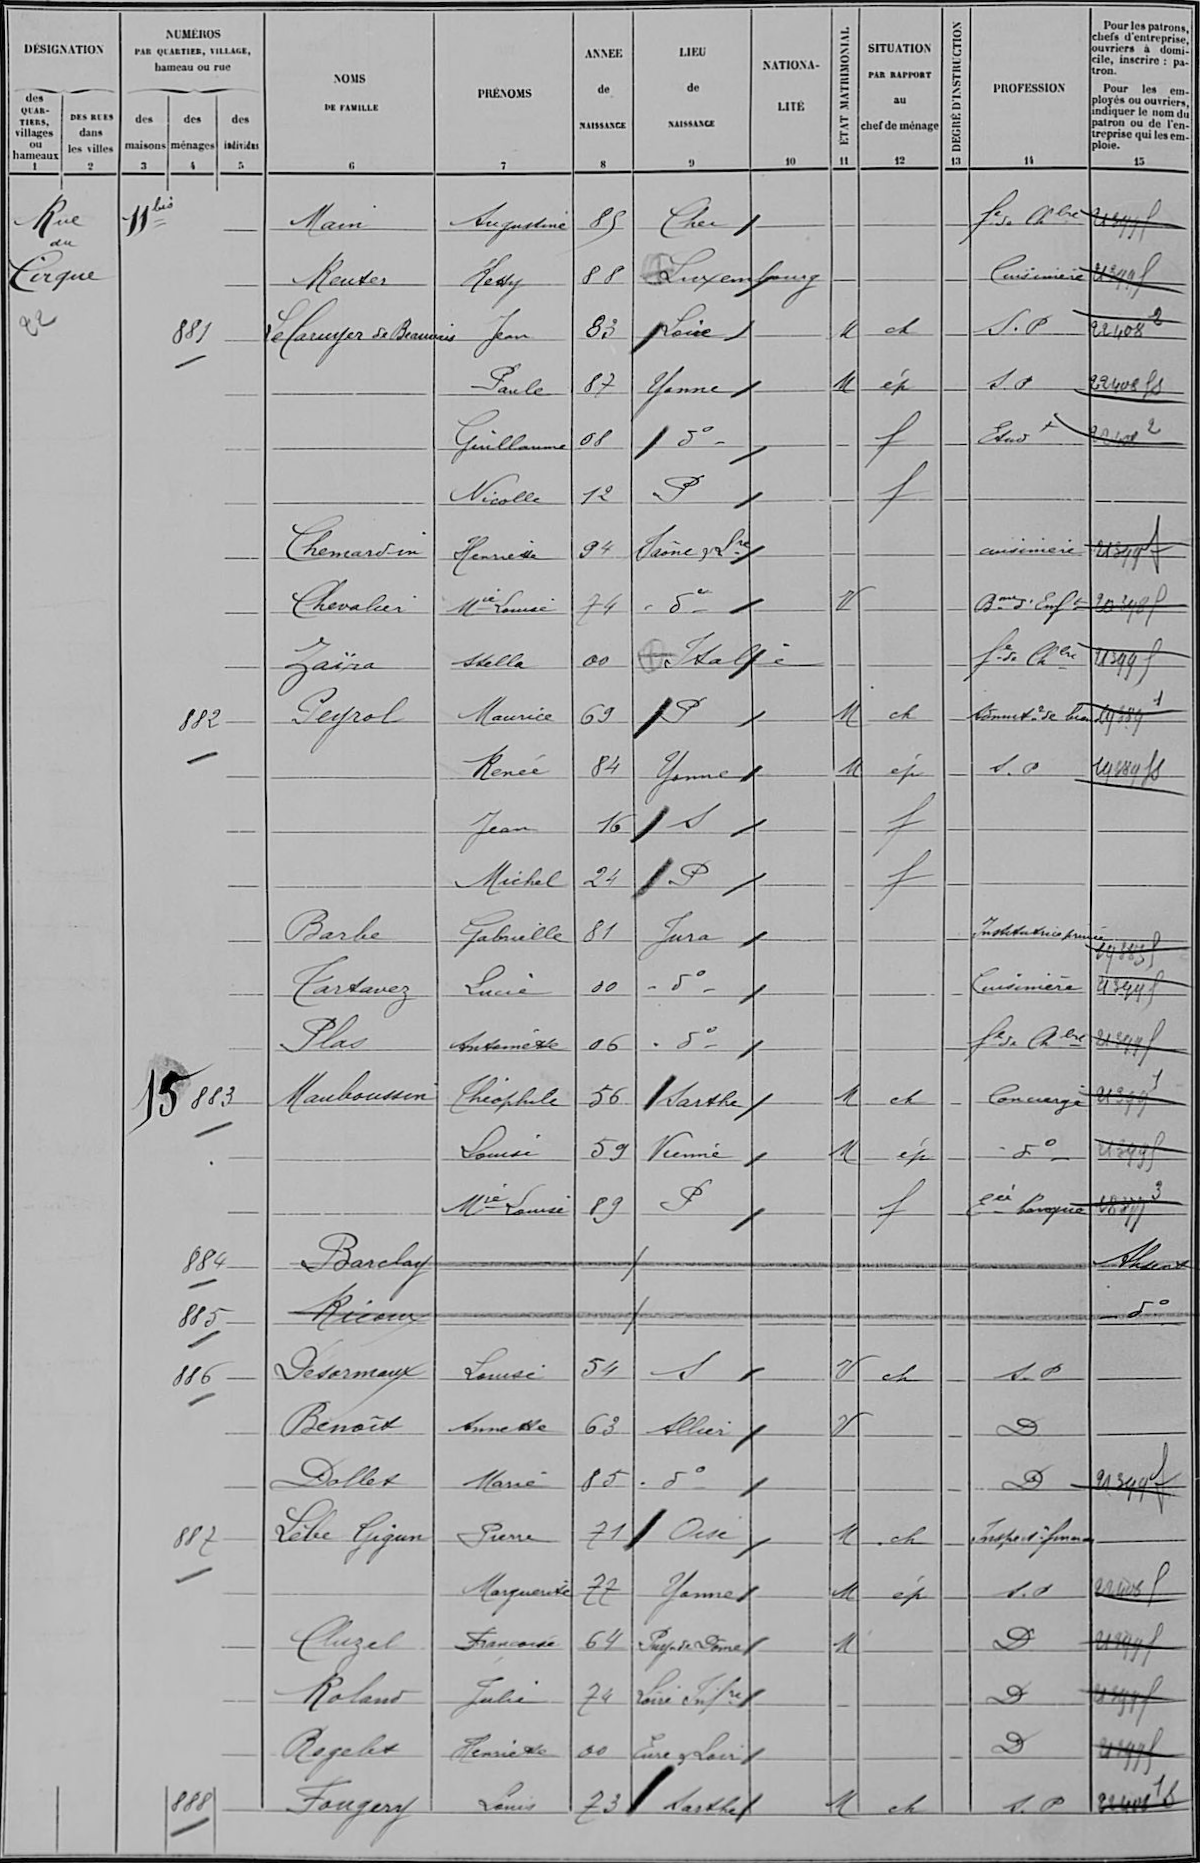Main/Augustine/85/Cher/¤/¤/¤/¤/fe de Chbre/21399 f
Reuter/Kessy/88/Luxembourg/¤/¤/¤/¤/Cuisinière/21399
Le Caruye de Beauvais/Jean/83/Loire/¤/M/ch/¤/S P/22408
¤/Paule/87/Yonne/¤/M/ép/¤/S P/22408 fs
¤/Guillaume/08/S°/¤/¤/f/¤/Etudt/22408
¤/Nicolle/12/P/¤/¤/f/¤/¤/¤
Chemardin/Henriette/94/Saône et Lre/¤/¤/¤/¤/cuisinière/21399 f
Chevalier/Mie Louise/74/d°/¤/V/¤/¤/Bne d'Enft/20348 f
Zaïra/Stella/00/Italie/¤/¤/¤/¤/fe de Chbre/21399 f
Peyrol/Maurice/69/P/¤/M/ch/¤/Bonnetr de bain/19389
¤/Renée/84/Yonne/¤/M/ép/¤/S P/19389 fs
¤/Jean/16/S/¤/¤/f/¤/¤/¤
¤/Michel/24/P/¤/¤/f/¤/¤/¤
Berbe/Gabrielle/81/Jura/¤/¤/¤/¤/Institutrice privée/19385
Tartavez/Lucie/00/d°/¤/¤/¤/¤/Cuisinière/21399 f
Plas/Antoinette/06/d°/¤/¤/¤/¤/fe de Chbre/21399 f
Mauboussin/Théophile/56/Sarthe/¤/M/ch/¤/Concierge/21399
¤/Louise/59/Vienne/¤/M/ép/¤/d°-/21399 f
¤/Mie Louise/89/P/¤/¤/f/¤/Eée banque/18377
Barclay/¤/¤/¤/¤/¤/¤/¤/¤/Absent
Rioux/¤/¤/¤/¤/¤/¤/¤/¤/d°
Desormaux/Louise/54/S/¤/V/ch/¤/S P/¤
Benoît/Annette/63/Allier/¤/V/¤/¤/D/¤
Dollet/Marie/875/d°/¤/¤/¤/¤/D/21399
Lèbe Gigun/Pierre/71/Oise/¤/M/ch/¤/Inspectr finances/¤
¤/Marguerite/77/Yonne/¤/M/ép/¤/S P/22408 f
Chizel/Françoise/64/Puy de Dôme/¤/M/¤/¤/D/21399 f
Roland/Julie/74/Loire Inf re/¤/¤/¤/¤/D/21399 f
Rogelet/Henriette/00/Eure & Loir/¤/¤/¤/¤/D/21399 f
Fougery/Louis/73/Sarthe/¤/M/ch/¤/S P/22408 f
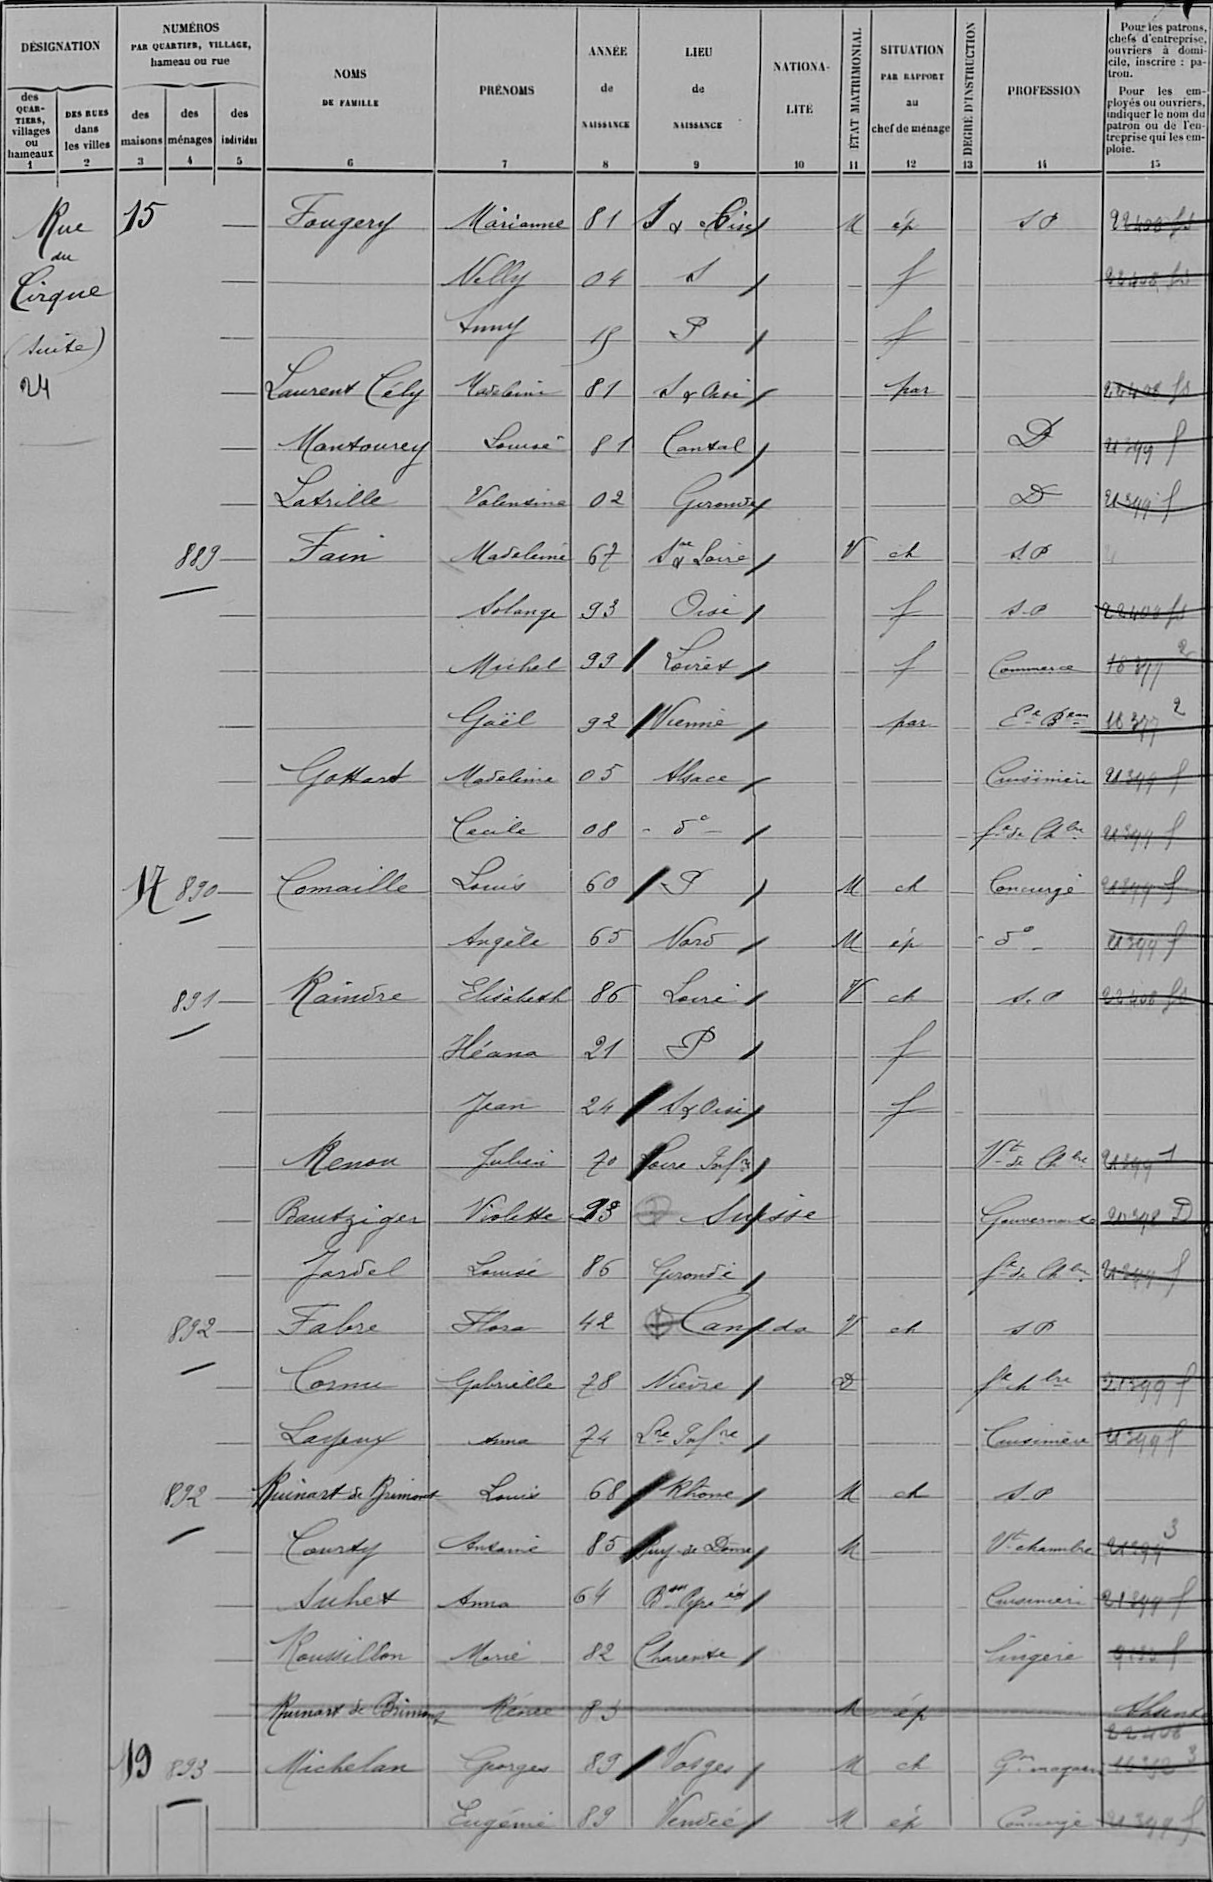Fougery/Marianne/81/S & Oise/¤/M/ép/¤/SP/22408fs
¤/Nlly/04/S/¤/¤/f/¤/¤/22408 fs
¤/Anny/15/P/¤/¤/f/¤/¤/¤
Laurent Cély/Madeleine/81/S&Oise/¤/¤/par/¤/¤/22408 fs
Mantourey/Louise/81/Cantal/¤/¤/¤/¤/D/21399 f
LAtrille/Valentine/02/Gironde/¤/¤/¤/¤/D/21399 f
Fain/Madeleine/67/Sne & Loire/¤/V/ch/¤/SP/¤
¤/Solange/92/Oise/¤/¤/f/¤/S P/22408 fs
¤/Michel/93/Loiret/¤/¤/f/¤/Commerce/18377
¤/Gaël/92/Vienne/¤/¤/par/¤/Ee Beau/18377
Gossaret/Madeleine/05/Alsace/¤/¤/¤/¤/Cuisinière/21399 f
¤/Cécile/08/d°/¤/¤/¤/¤/fe de Chbre/21399 f
Comaille/Louis/60/P/¤/M/ch/¤/Concierge/21399 f
¤/Angèle/65/Nord/¤/M/ép/¤/d°/21399 f
Raindre/Elisabeth/86/Loire/¤/V/ch/¤/S P/22408 fs
¤/Iléana/21/P/¤/¤/f/¤/¤/¤
¤/Jean/24/S&Oise/¤/¤/f/¤/¤/¤
Renou/Julien/70/Loire Infre/¤/¤/¤/¤/Vt de Chbre/21399
Bautziger/Violette/93/Suisse/¤/¤/¤/¤/Gouvernante/20398 D
Jardel/Louise/86/Gironde/¤/¤/¤/¤/fe de Chbre/21399 f
Fabre/Flora/42/Canada/¤/V/ch/¤/SP/¤
Cornu/Gabrielle/78/Nièvre/¤/D/¤/¤/fe chbre/21399 f
Lageux/anna/74/Lre Infe/¤/¤/¤/¤/Cuisinière/21399 f
Ruinard de Brimont/Louis/68/Rhône/¤/M/ch/¤/S P/¤
Courty/Antoine/85/Puy de Dôme/¤/M/¤/¤/Vt chambre/21399
Suchet/Anna/64/Basses Pyreées/¤/¤/¤/¤/Cuisinière/21399 f
Roussillon/Marie/82/Charente/¤/¤/¤/¤/lingère/9133 f
Ruinart de Brimont/Renée/83/¤/¤/M/ép/¤/¤/Absente!22408
Michelan/Georges/89/Vosges/¤/M/ch/¤/Gon magasin/16352
¤/Eugénie/89/Vendée/¤/M/ép/¤/Concierge/21399 f